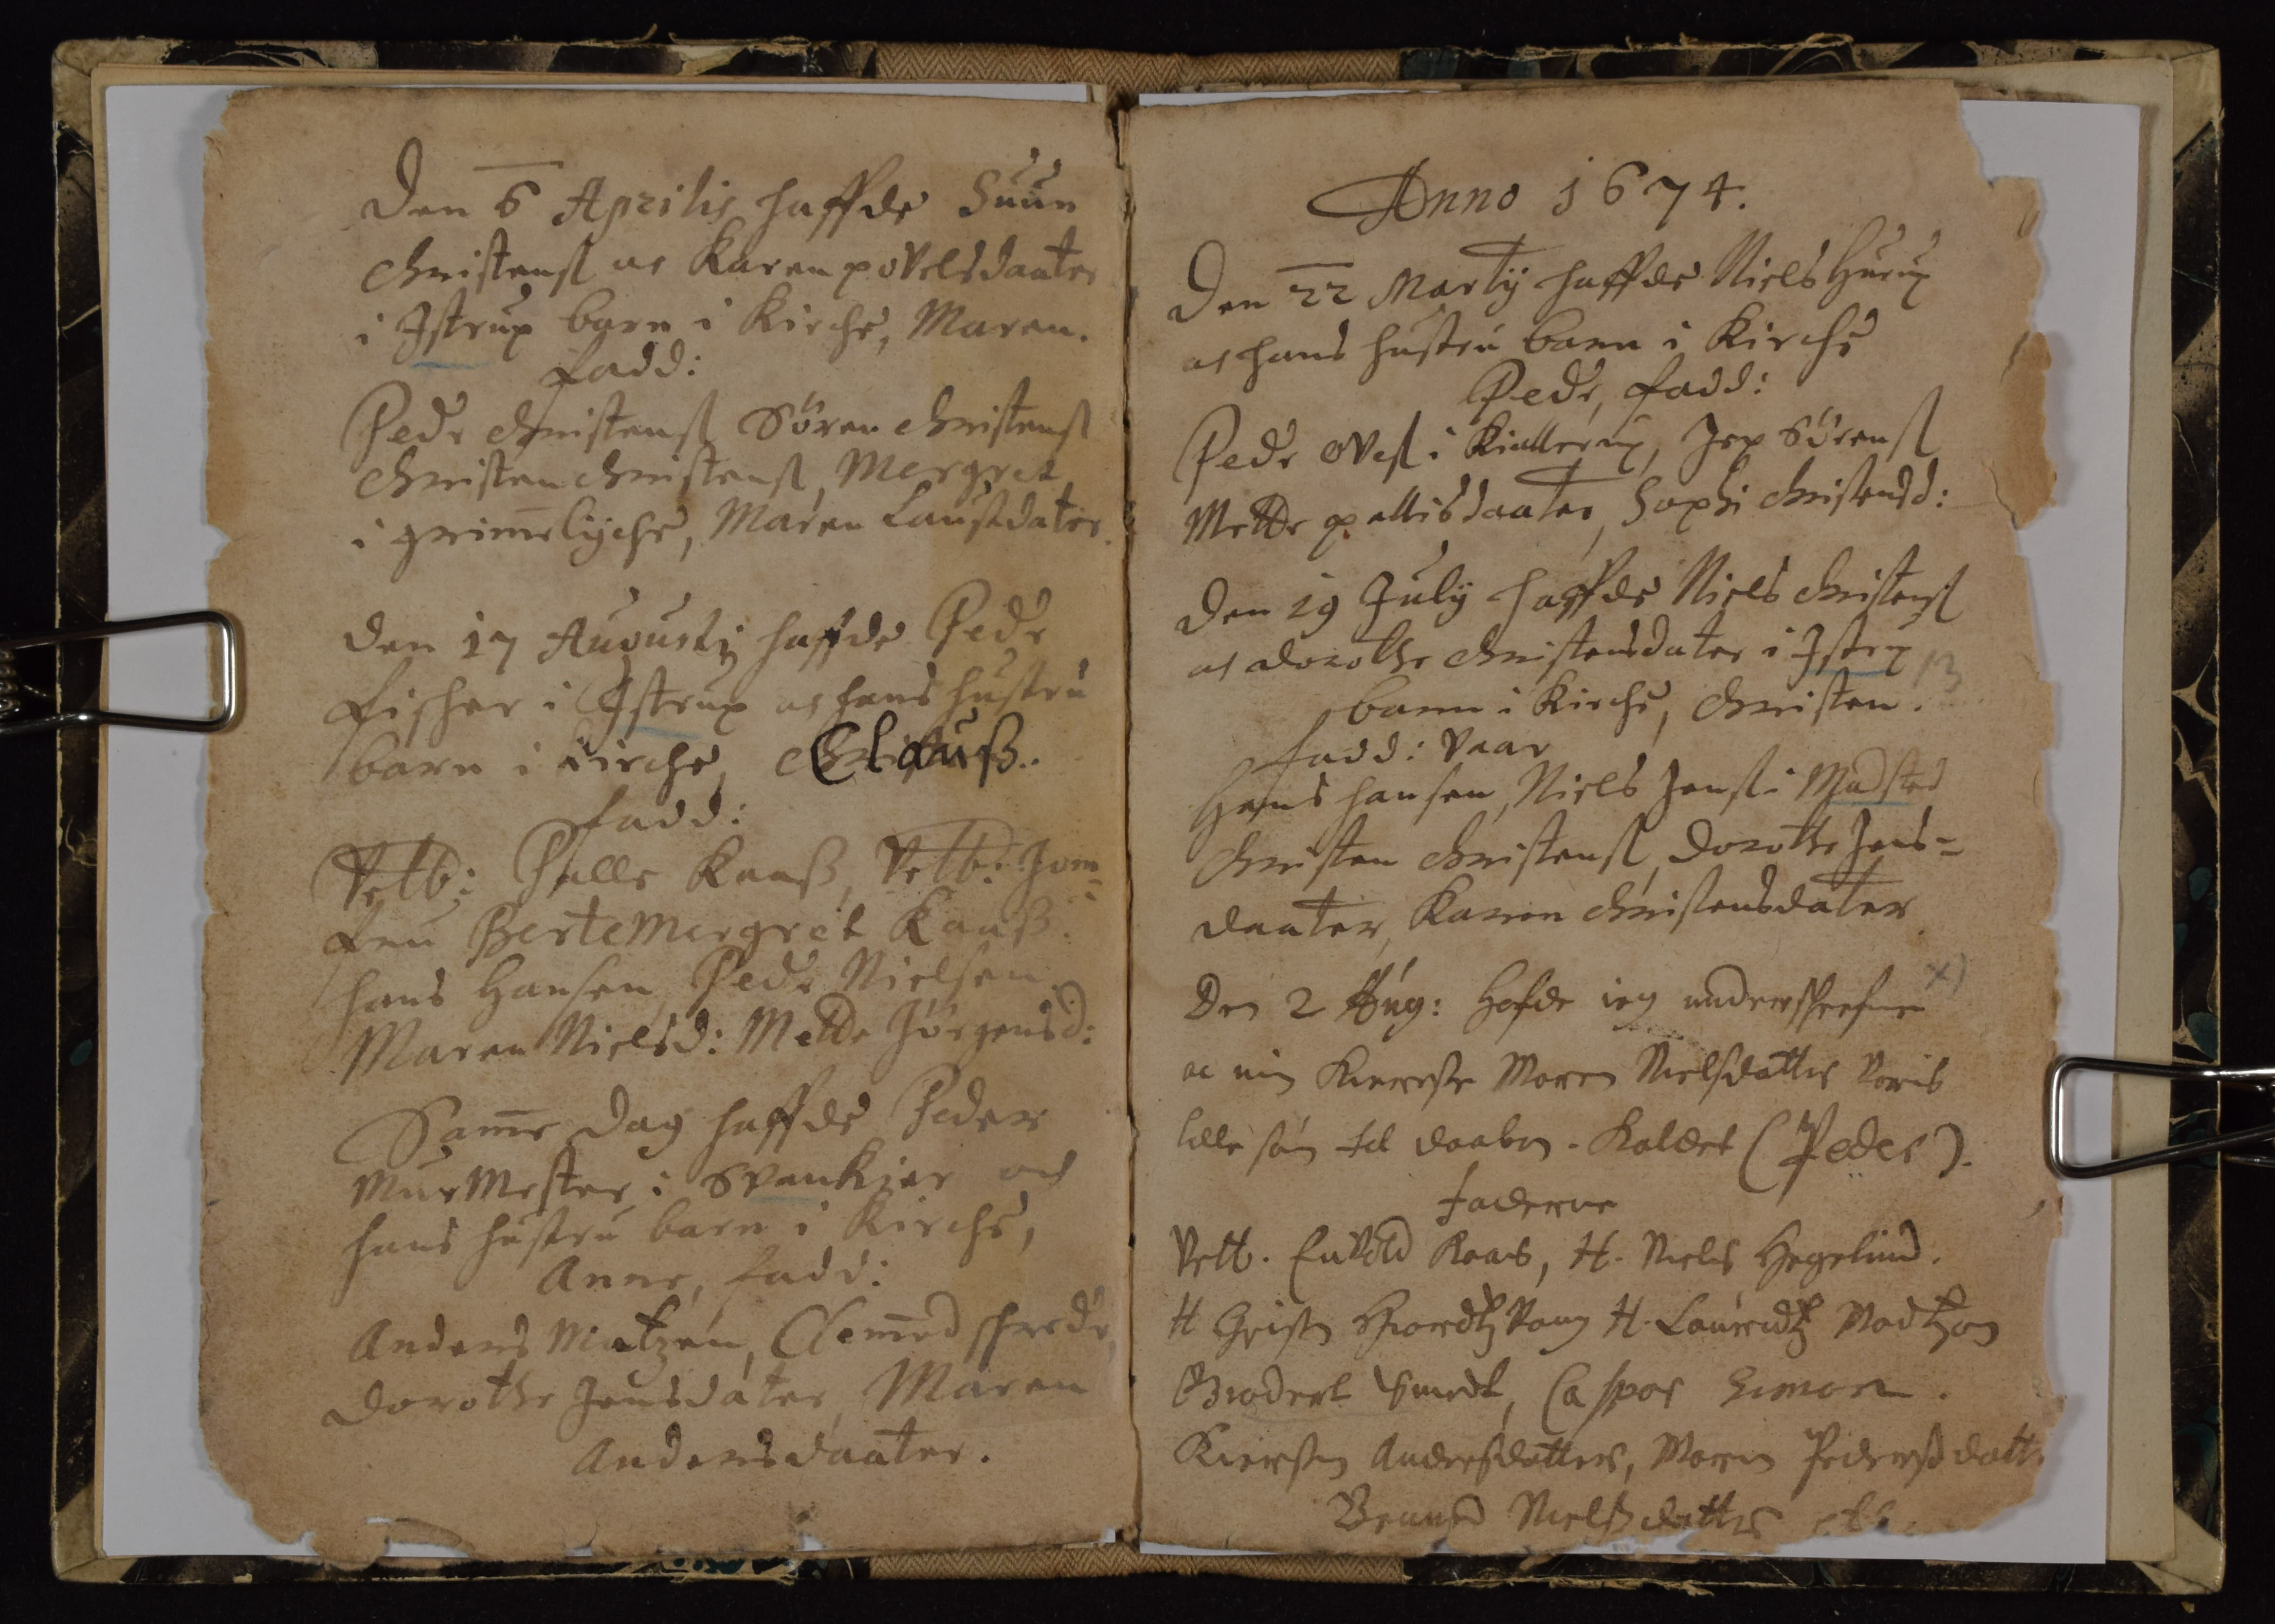Den 6 Aprilis haffde Suun
Christens oc Karen povelsdaater
i Istrup barn i Kirche, Maren
Fadd:
Pedr christens Søren christens
christen christens Mergret
i Grimelyche, Maren Laustdater
Den 17 Augustij haffde Pedr
Fisher i Istrup och hans hustru
barn i Kirche Clauss.
Fadd:
Velb: Palle Kaass Velb: Jom¬
fru Berte Mergret Kaass.
hans Hansen Pedr Nielsen
Maren Nielsd: Mette Jørgensd:
Samme dag haffde Peder
Mur Mester i Svankier och
hans hustru barn i Kirche,
Anne; Fadd:
Anders Matzen, Clemmed shrede,
Dorothe Jensdater, Maren
Andersdaater.
Anno 1674.
Den 22 Martij haffde Niels Hueug
och hans hustru barn i Kirche
Pedr Fadd:
Pedr Oves i Kallerup, Jep Sørens
Mette pallisdaater Sophi christensd:
Den 19 Julij haffde Niels christens
och Dorothe christensdater i Istrup
barn Kirche, christen.
Fadd: vaar
Hans hansen Niels Jens i Madsted
Chirsten Christens Dorotte Jens¬
daater Karen christensdater
Den 2 Aug: hafde ieg undershrefue
oc min Kiereste Maren Nielsdatters voris
lille søn til Daaben - kaldet (Peder).
Faderne
Velb. Envold Kaas, H: Niels Hegelund
H Christ Hiordtz Vong H. Lauridtz Madtzon
Broderl Smedt, Caspor Simon.
Kiersten Andersdatter, Maren Pedersdatter
Brune Nielssdatter ##
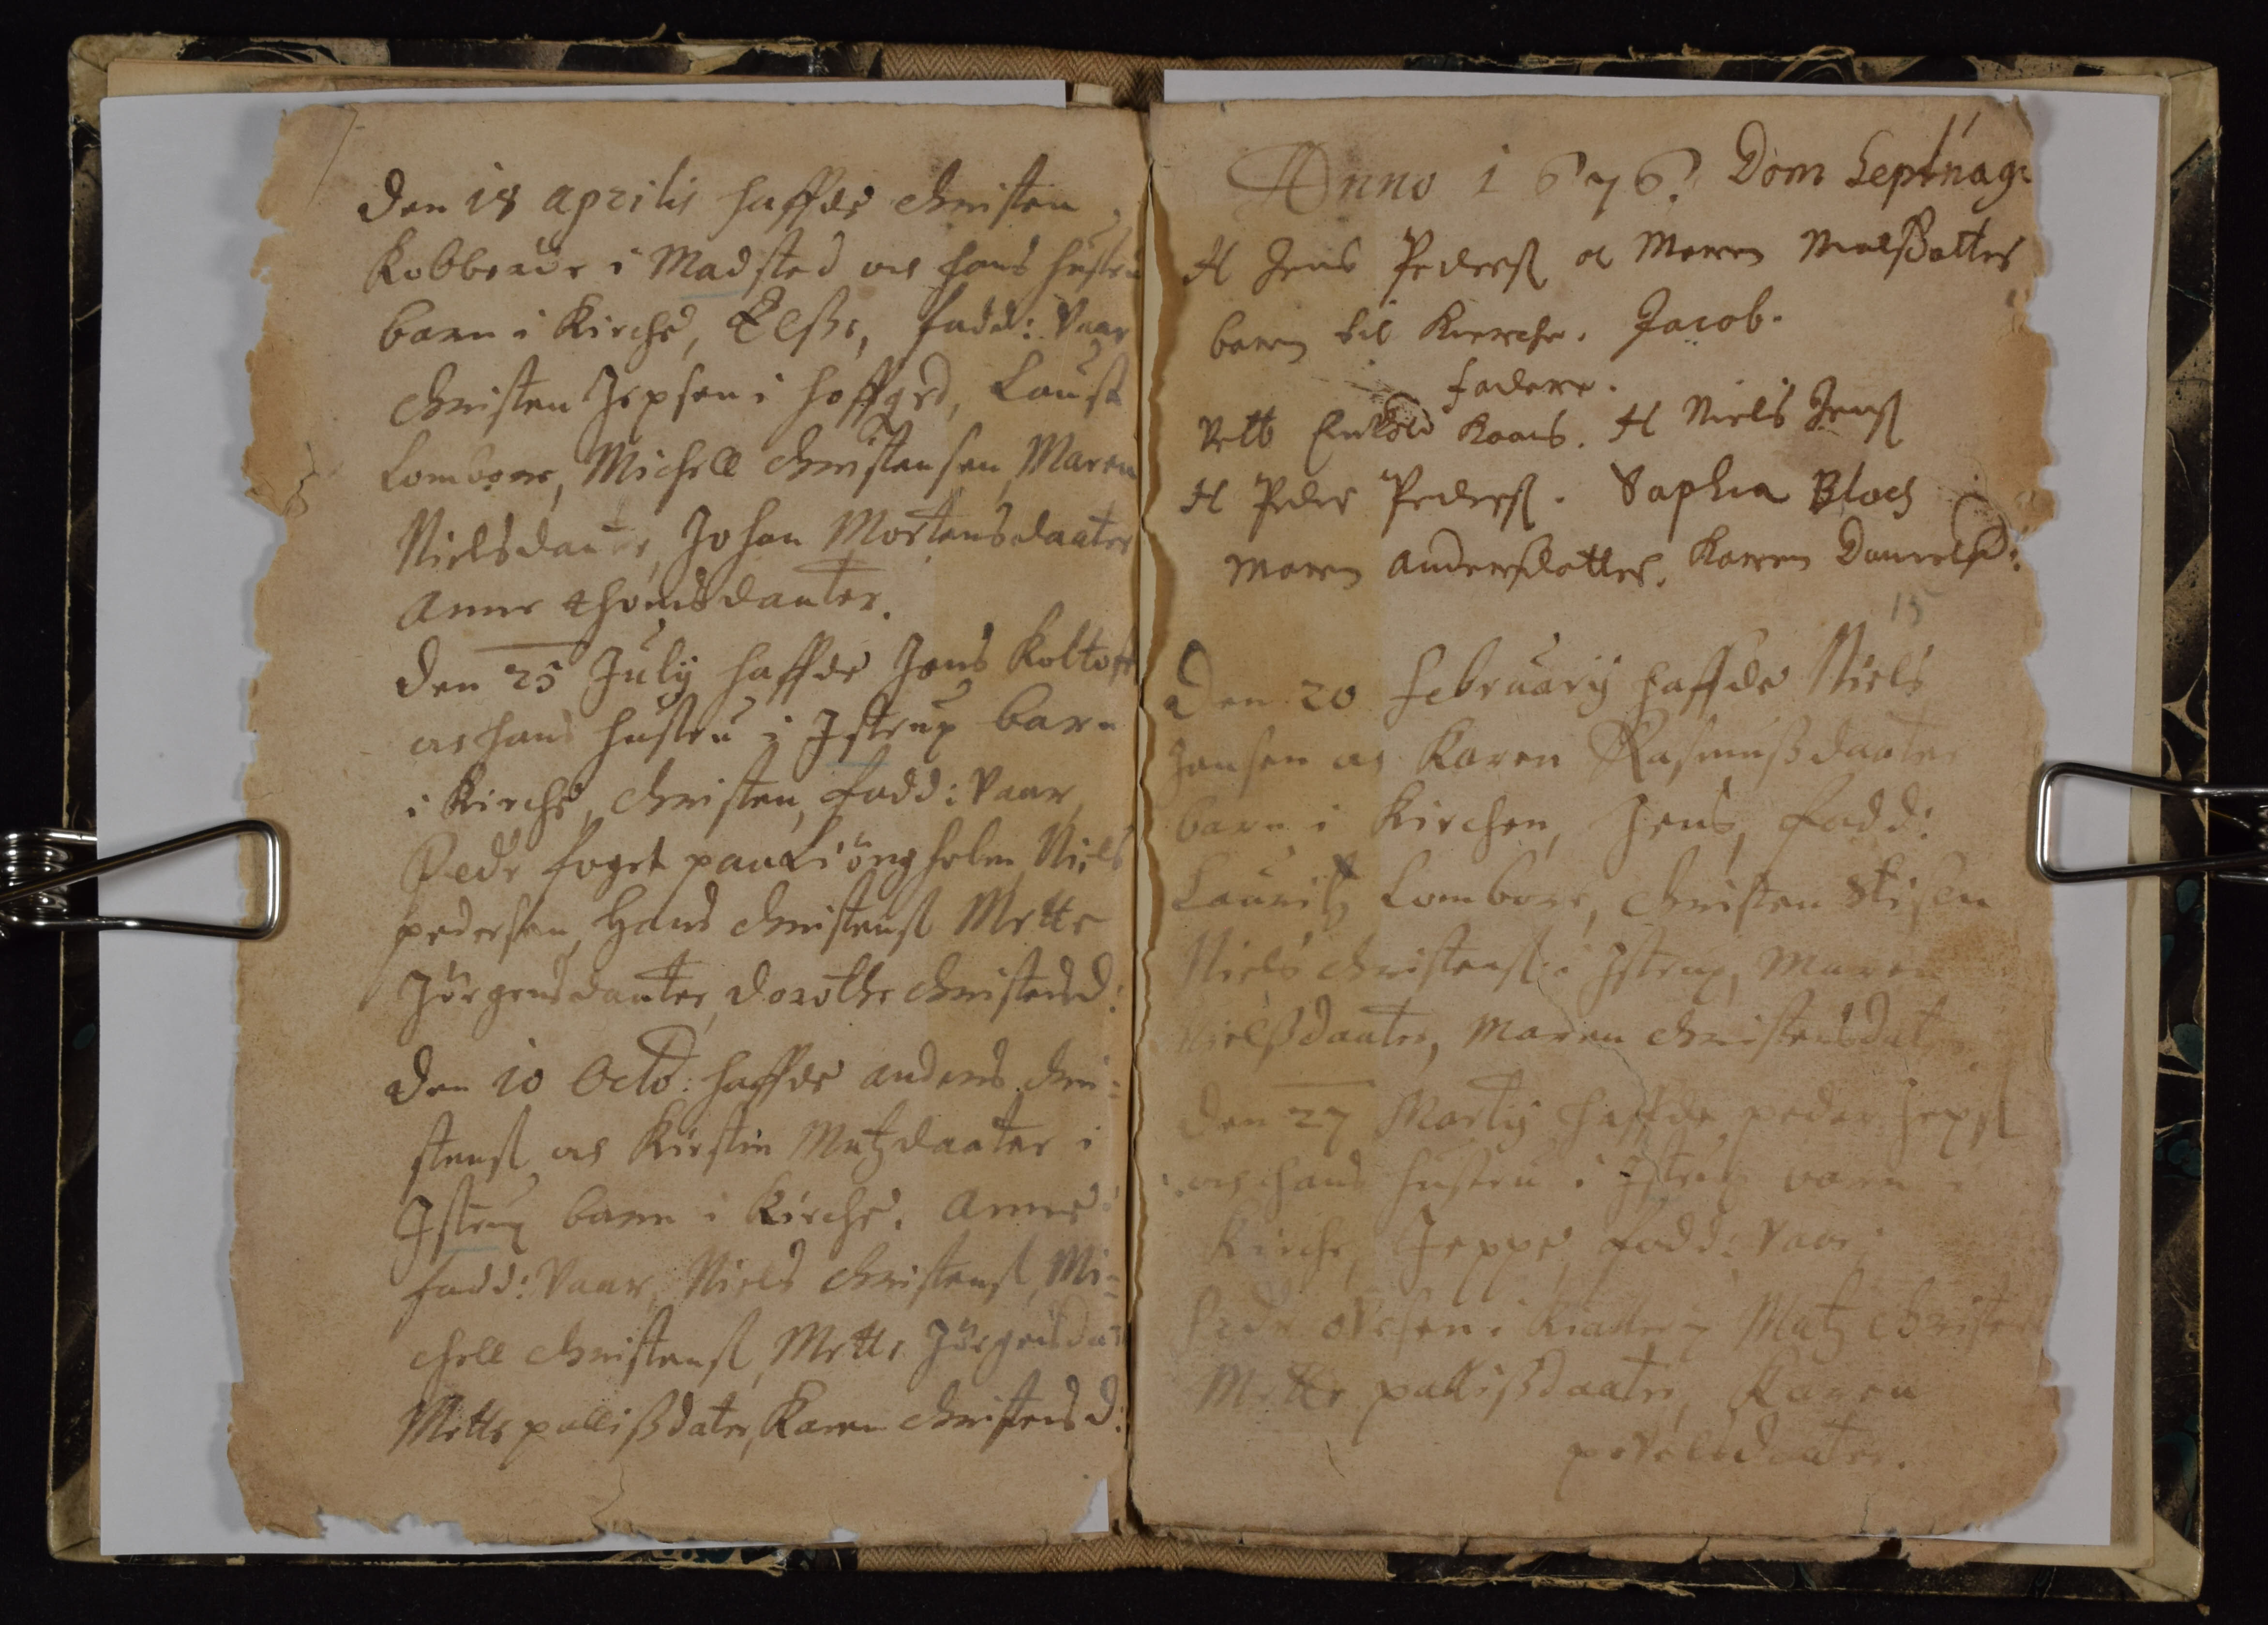Den 18 Aprilis haffde Christen
Kobbeaue i Madsted och hans hustru
barn i Kirche, Elsse. Fadd: vaar
Christen Jepsen i hoffgrd Laust
Lombone, Michell cChristensen Maren
Nielsdaater Johan Mortensdaater
Anne Thomsdauter
Den 25 Julij haffde Jens Koltoff
och hans hustru i Istrup barn
i Kirche christen Fadd: vaar
Pedr foget paa Liøngholm Niels
Pedersen Hans Christens Mette
Jørgensdaater Dorothe Christendsd:
Den 10 Octo: haffde Anders chri¬
stens och Kirstin Matzdaater i
Istrup barn i Kirche: Anne
Fadd: vaar; Niels christens Mi¬
chell Christens Mette Jørgensdat
Mette pallisdater Karen christensd:
Anno 1676 Dom Septuag:
H Jens Peders oc Maren Nielsdatter
barn til Kirche. Jacob.
Fadere
Velb Envold Kaas. H Niels Jens
H Peder Peders. Saphia Bloch
Maren Andersdatter, Karen Danielsd:
Den 20 Februarij haffde Niels
Jensen og Karen Rasmussdaater
barn i Kirchen, Jens; Fadd:
Lauritz Lombore, Christen Stisen
Niels christen i Istrup Maren
Nielsdaater, Maren Christensdatter
Den 27 Martij haffde peder Jeps
och Hans Hustru i Istrup barn i
Kirche Jeppe Fadd: vaar
Pedr ovesensi Kiallerup Matz christens
Mette pallissdaater Karen
povelsdaater